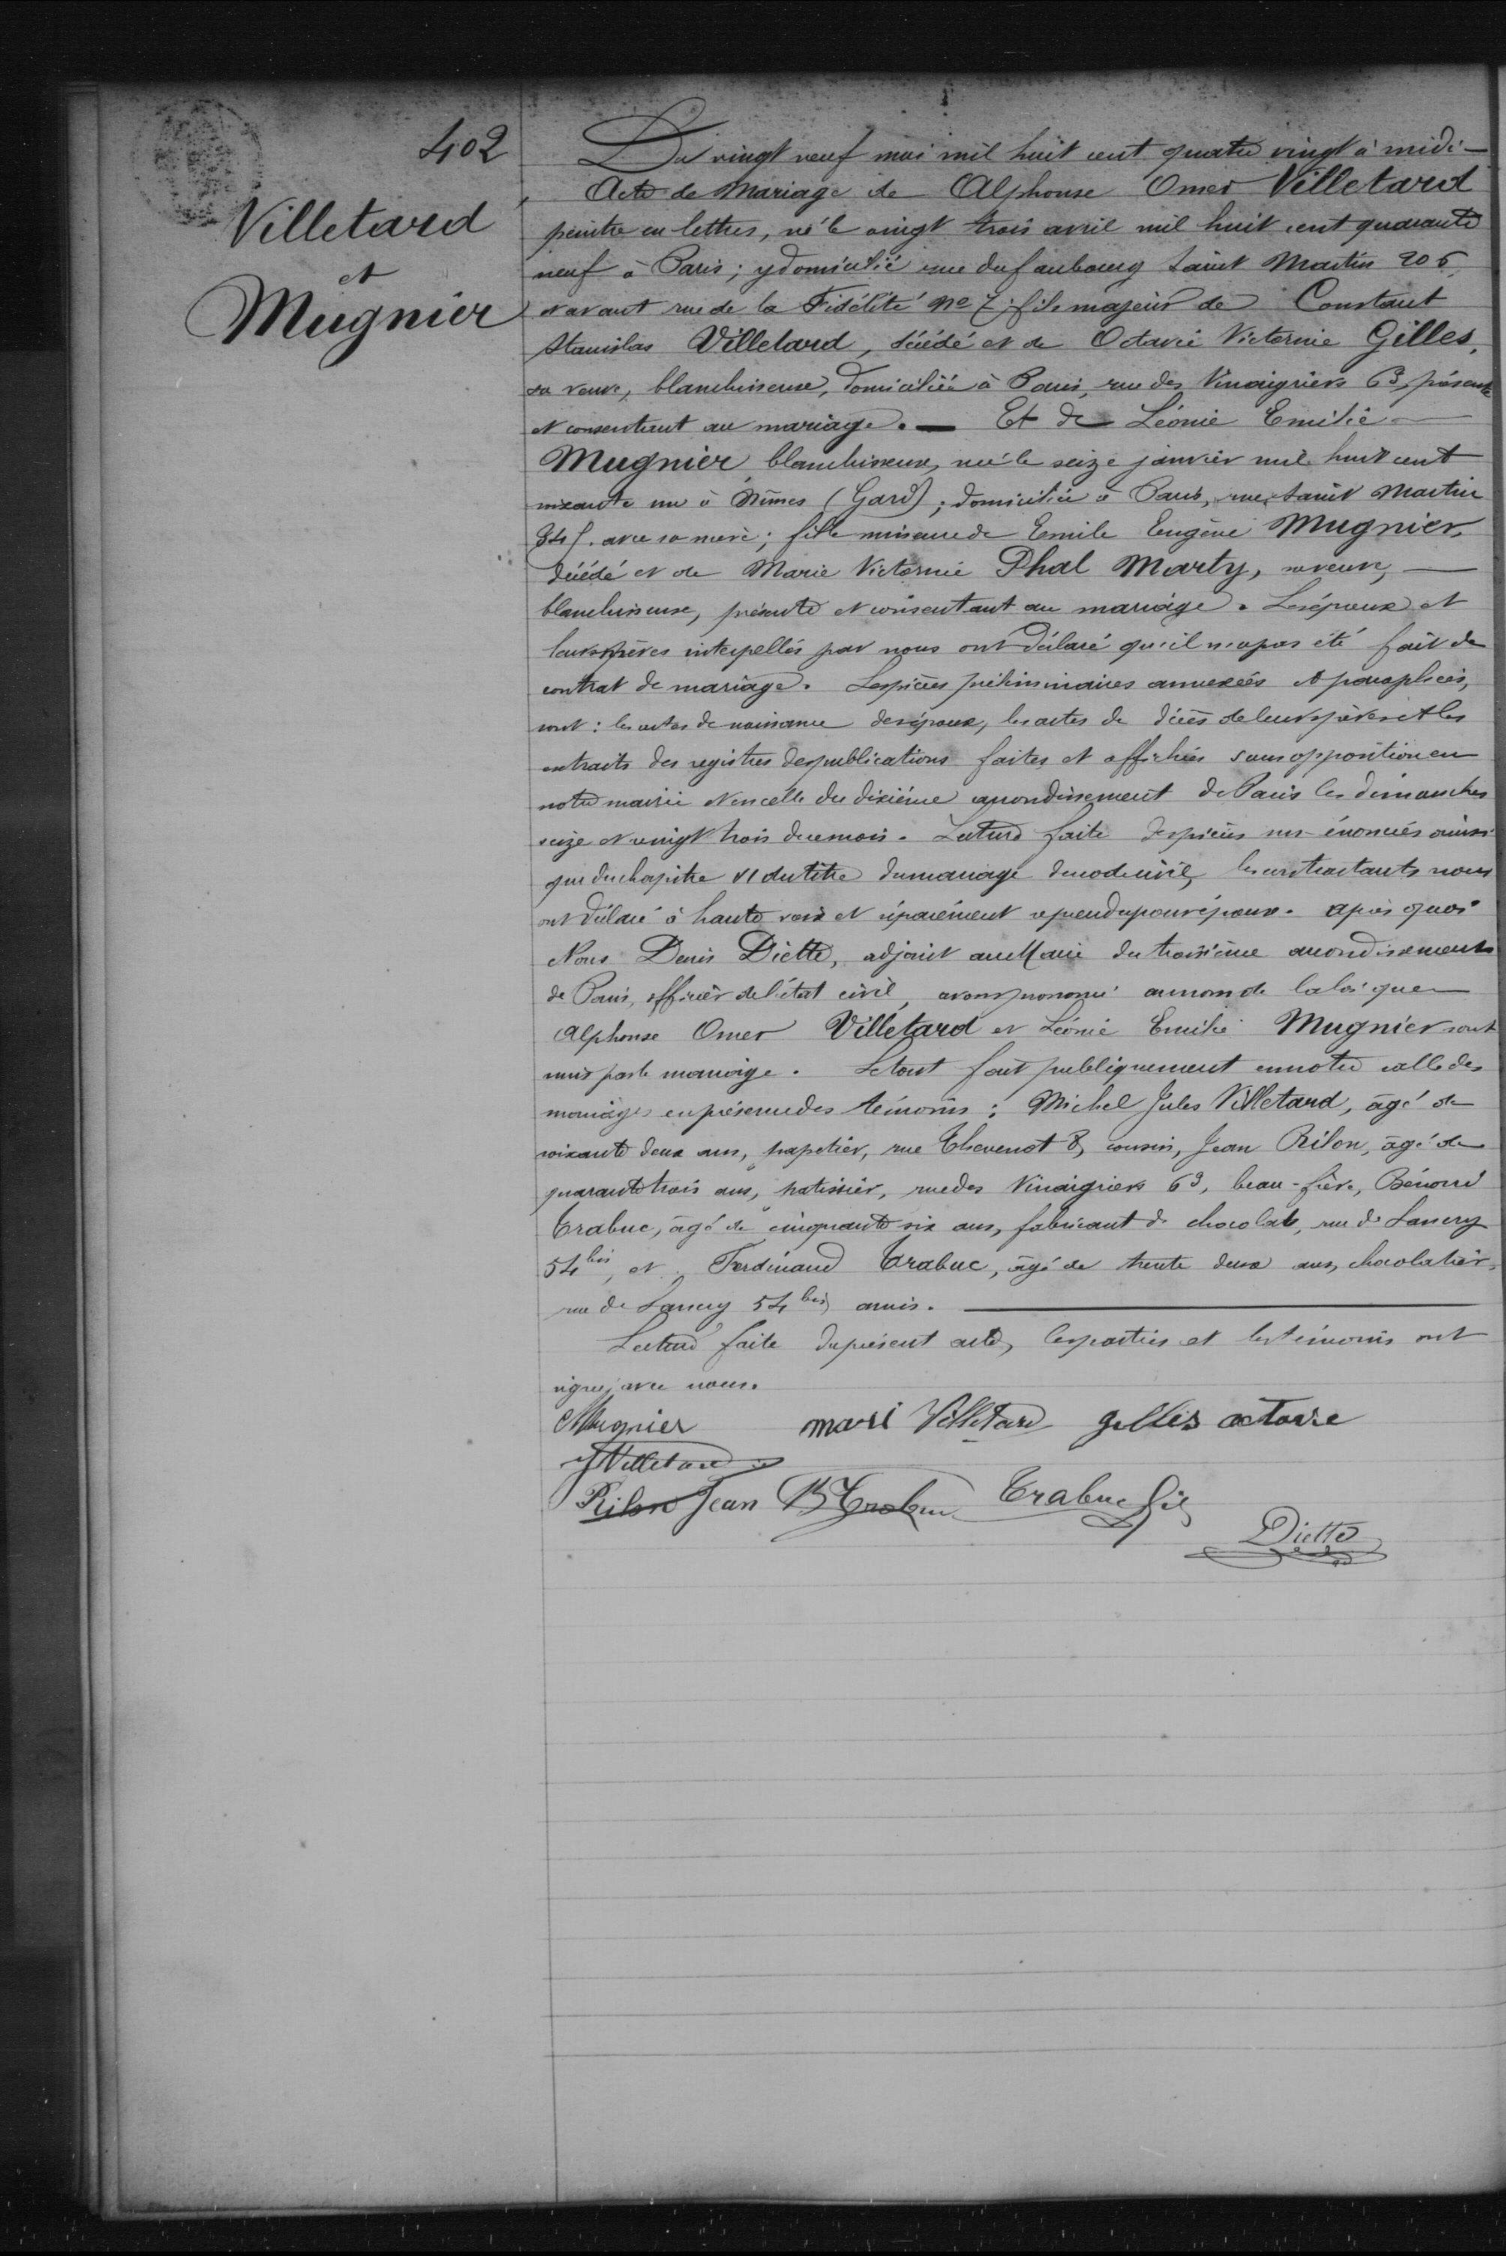402
Villetard
et
Mugnier
Du vingt neuf mai mil huit cent quatre vingt à midi-
Acte de Mariage de Alphonse Omer Villetard
peintre en lettres, né le vingt trois avril mil huit cent quarante
neuf à Paris; y domicilié rue du faubourg Saint Martin 205
et avant rue de la Fidelité n°7 fils majeur de Constant
Stanislas Villevard, décédé et de Octavi Victorine Gilles
sa veuve, blanchisseuse, domicilée à Paris, rue des Vinaigriers 63, présente
et consentant au mariage. -Et de Léonie Emilie
Mugnier blanchisseuse, née le seize janvier mil huit cent
soixante un à Nîmes (Gard); domicilié à Paris, rue Saint Martin
34, avec sa mère; fille mineure Emile Eugène Mugnier,
décédé et de Marie Victorine Phal Marty, sa veuve
blanchisseuse, présente et consentant au mariage. Les époux et
leurs mères interpellées par nous ont déclaré qu'il n'a pas été fait de
contrat de mariage. Les pièces préliminaire annexées et paraphées,
sont: les actes de naissance des époux, les acte de décès de leur père et les
extraits des registres de publications faites et affichée sans opposition en
notre mairie et en celle du dixème arrondissement de Paris les dimanches
seize et vingt trois du mois. Lecture faite des pièces sus-énoncés ainsi
que du chapitre VI du titre du mariage du code civil, les contractants nous
ont déclaré à haute voix et séparément se prendre pour époux. Après quoi
Nous Denis Diette, adjoint au Maire du troisième arrondissement
de Paris, officier de l'état civil, avons prononcé au nom de la loi que
Alphonse Omer Villetard et Léonie Emilie Mugnier sont
unis par le mariage. Le tout fait publiquement en notre salle des
mariage en présence des témoins: Michel Jules Villetard, âgé de
soixante deux ans, papetier, rue Chevenot 8, cousin Jean Prillon, âgé de
quarante trois ans, patissier, rue des Vinaigriers 69, beau-frère, Bénond
Trabuc, âgé de cinquante six ans, fabricant de chocolat, rue de Lancry
54 bis, et Ferdinand Trabuc, âgé de trente deux ans, chocolatier
rue de Lancy 57 bis amis.-
Lecture faite du présent acte, les parties et les témoins ont
signé avec nous.
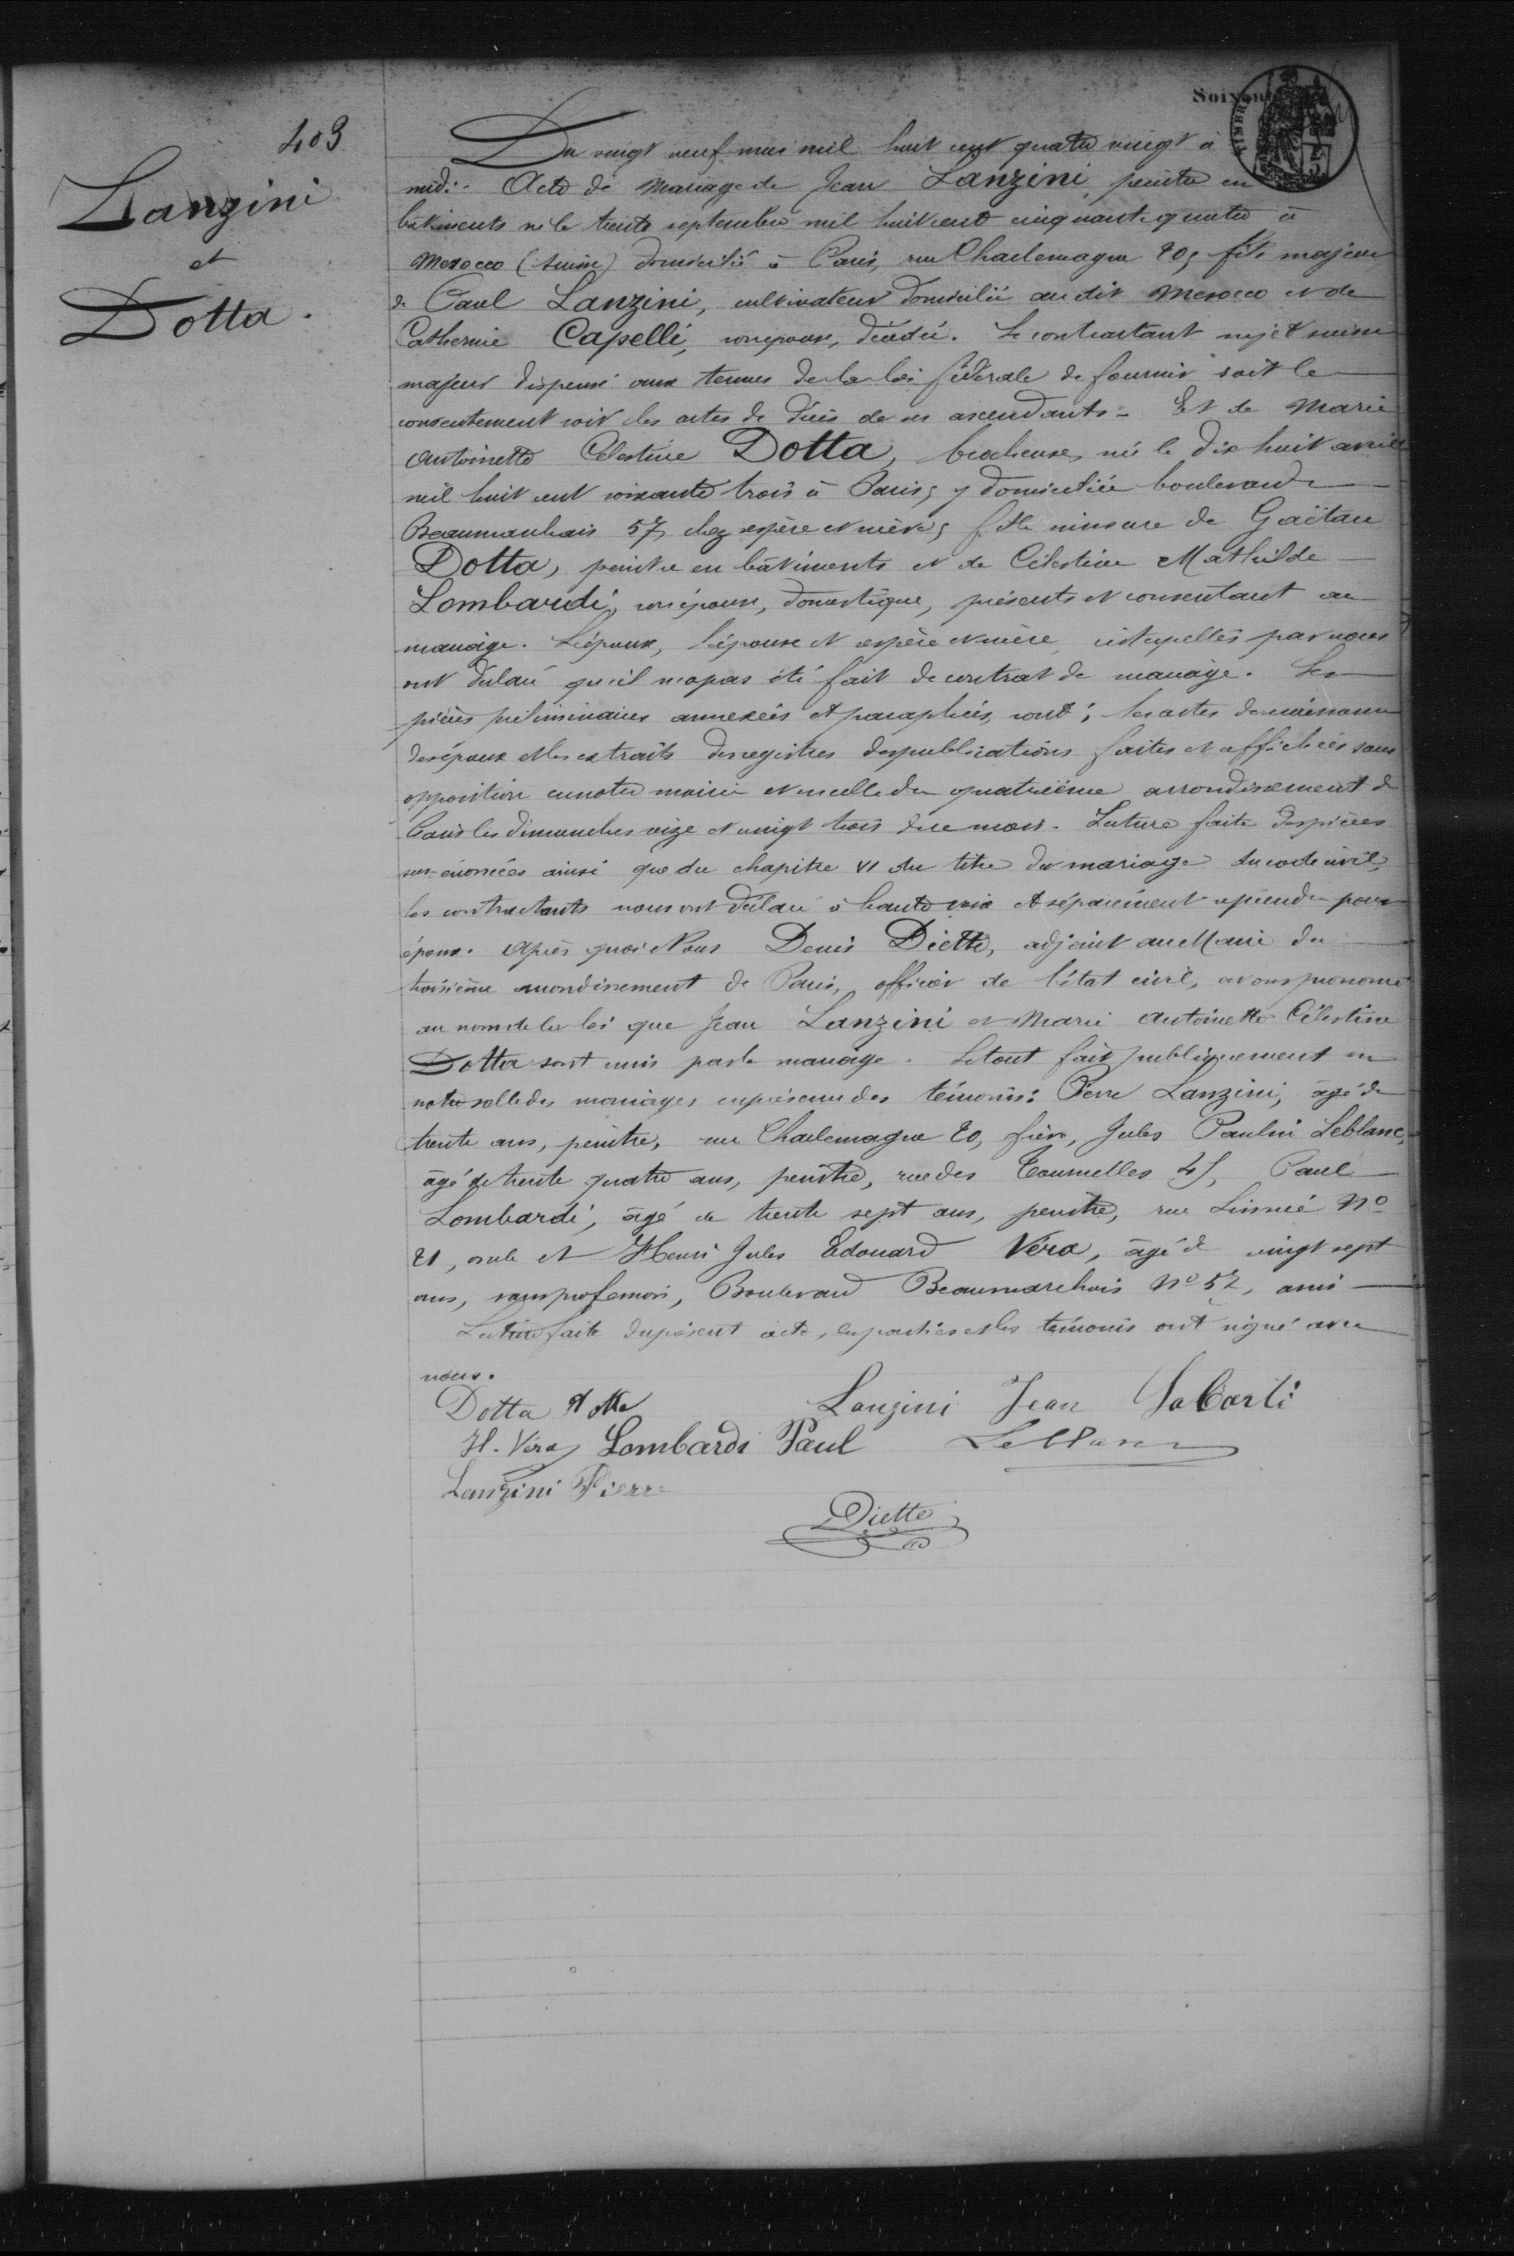403
Langini
et
Dotta
Du vingt neuf mai mil huit cent quatre vingt à
midi Acte de mariage de Jean Lanzini peintre en
bâtiments ne le treize septembre mil huit cent cinquante quatres à
Merocco (Seine) domicilié à Paris, rue Chalemadue, 205 fils majeur
de Paul Lanzini, cultivateur domicilié au dit Merocco et de
Catherine Capellé, son épouse, décédée. Le contractant agé de vingt
majeur dispensé aux tenues de la loi déférale de fournit soit le -
consentement voit des actes des décès de ses ascendantes -Et de Marie
Antoinette Celestine Dotta, brocheuse, née le dix-huit avril
mil huit cent soixante trois à Paris, y domiciliée boulevard -
Beaumarchais 57, chez ses père et mère, fille mineure de Gaëtan
Dotta, peintre en bâtiments et de Célestine Mathilde -
Lombardi, son épouse, domestique, présents et consentant au
mariage. L'épouse, l'époux et père et mère interpellés par nous
ont déclaré qu'il n'a pas été fait de contrat de mariage. Les
pièces préliminaire annexées et paraphées, sont; les actes de naissance
des époux et les extraits des registres de publication faite et affichées sans
opposition en notre mairie et celle du quatrième arrondissement de
Paris les dimanche seize et vingt trois mars. Lecture faite despièces
sus énoncées ainsi que du chapitre VI du titre du mariage du code civil
les contractants nous ont déclaré à haute voix et séparément se prendre pour
époux. Après quois Nous Denis Diette, adjoint au Maire du
troisième arrondissement de Paris, officier de l'état civil, avons prononcé
au nom de la loi que Jean Lanzini et Marie Antoinette Célestine
Dotta sont unis par le mariage. Letout fait publiquement en
notre salle des mariages en présence des témoins: Pierre Lanzini, âgé de
trente ans, peintre, rue Charlemagne 20, frère, Jules Paulini Leblanc,
âgé de trente quatre ans, peintre, rue des Tourmelles 41, Paul -
Lombardi, âgé de trente sept ans, peintre, rue Linné N°
21, oncle et Henri Jules Edouard Véra, âgé de vingt sept
ans, sans profession, Boulevard Beaumarchais N°52, amis -
Lecture faite du présent acte, les parties et les témoins ont signé avec
nous.